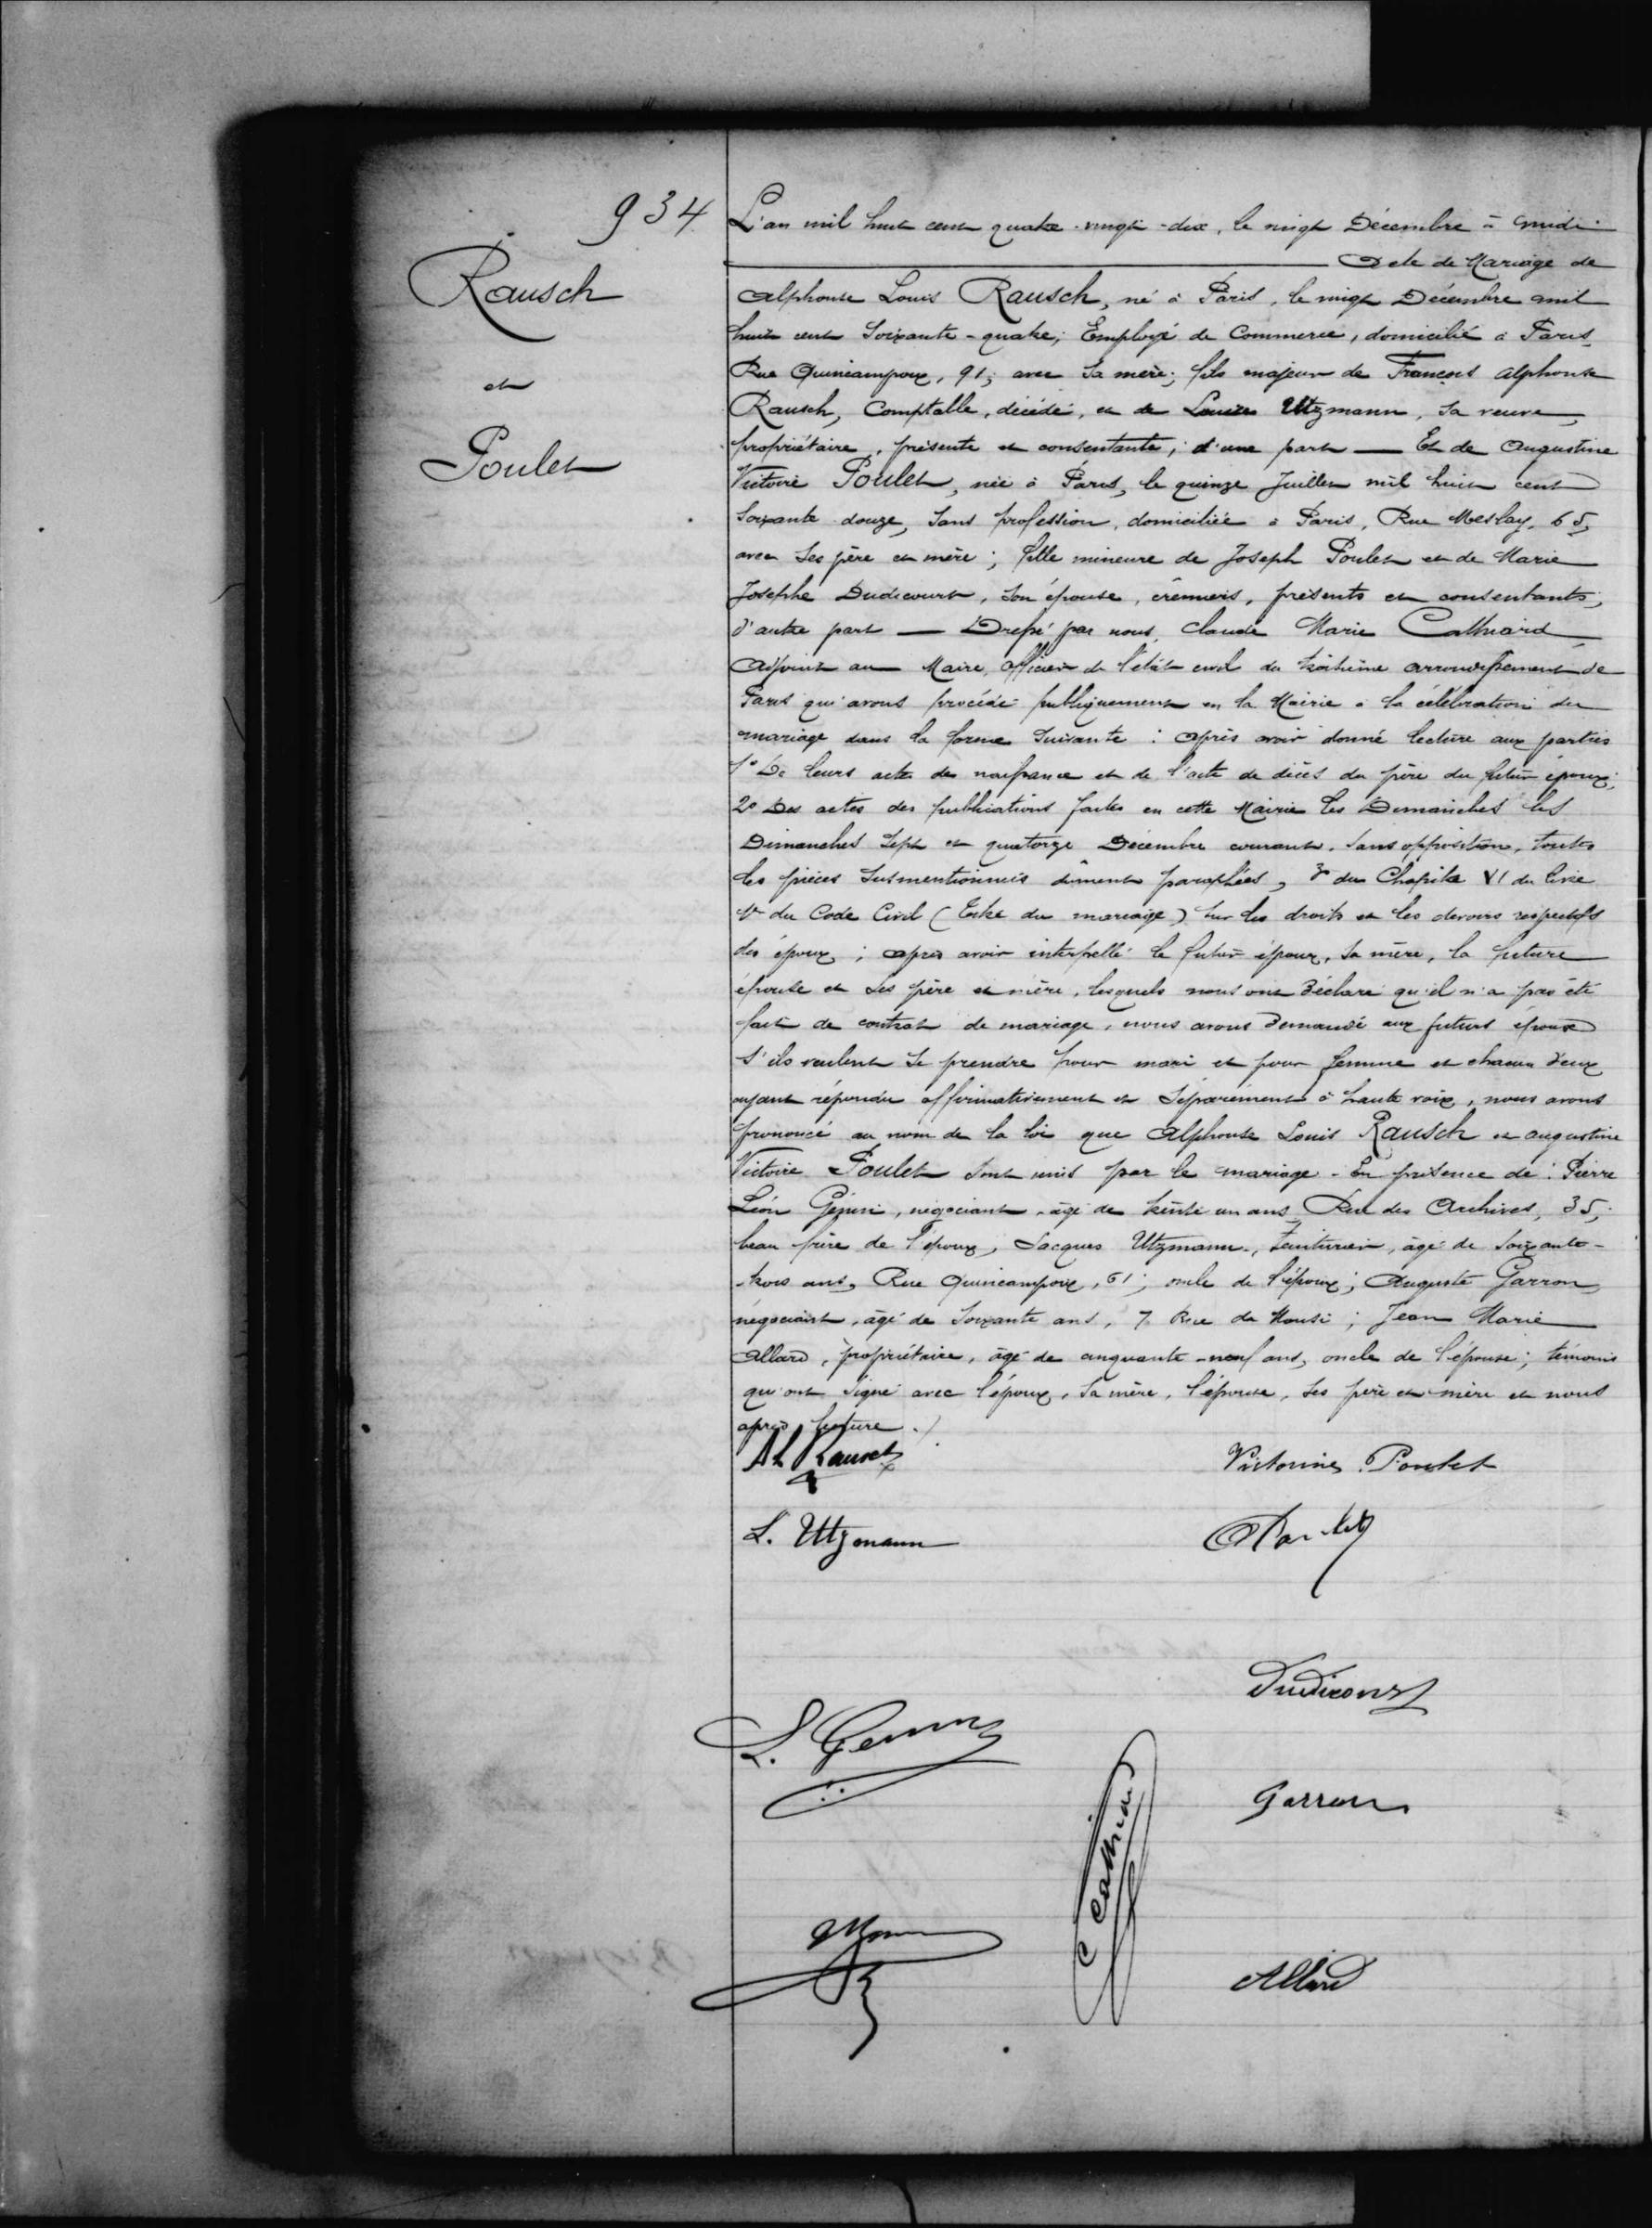934
Rausche
et
Poulet
L'an mil huit cent quatre ving-deux, le vingt Décembre à midi
-Acte de Mariage de
Alphonse Louis Rausch, né à Paris, le vingt Décembre mil
huit cent Soixante-quatre; Employé de Commerce, domicilié à Paris,
Rue Quincampoix, 91, avec sa mère; fils majur de Froment alphonse
Rausch, Comptable, décédé, et de Louise Ultzmann, sa veuve,
propriétaire, présente et consentante; d'une part-Et de Augustin
Victoire Poulet, née à Paris, le quinze Juillet mil huit cent
soixante douze, sans profession, domiciliée à Paris, Rue Meslay, 65,
avec ses père et mère; fille mineure de Joseph Poulet et de Marie
Josepha Dudecourt, son épouse, crêmiers, présents et consentants
d'autre part -Dressé par nous, Claude Marie Cathiard,
Adjoint au Maire, officier de l'état civil du troisième arrondissement de
Paris qui avons procédé publiquement en la Mairie. la célébration du
mariage dans la forme suivante: après avoir donné lecture aux parties
1° De leurs actes de naifsance et de l'acte de décès du père du futur époux
2° Des actes des publications faites en cette Mairie les Dimanches les
Dimanches sept et quatorze Décembre courant. Sans oppositions, toutes
les pièces susmentionnées dûment paraphées, 3° du Chapitre VI du livre
V du Code Civil (Titre du mariage) sur les droits et les devoirs respectifs
des époux; après avoir interpellé le futur époux, sa mère, la futur
épouse et ses père et mère, lesquels nous ont déclaré qu'il n'a pas été
fait de contrat de mariage, nous avons demandé aux futur époux
s'ils veulent se prendre pour mari et pour femme et chacun d'eux
ayant répondu affirmativement et séparèment à haute voix, nous avons
prononcé au nom de la loi que Alphonse Louis Rausch et augustine
Victoire Poulet sont unis par le mariage. En présence de: Pierre
Léon Génin, négociant, âgé de trente un ans Rue des Archives, 35;
beau père de l'époux, Jacques Uttzmam, teinturier, âgé de soixante
-trois ans, Rue Quincampoix, 61; oncle de l'époux; Auguste Garron
négociant, âgé de Soixante ans, 7 Rue de Mousi; Jean Marie
Allard, propriétaire, âgé de cinquante-neuf ans, oncle de l'épouse; témoins
qui ont signé avec l'époux, sa mère, l'épouse, ses père et mère et nous
après lecture./.
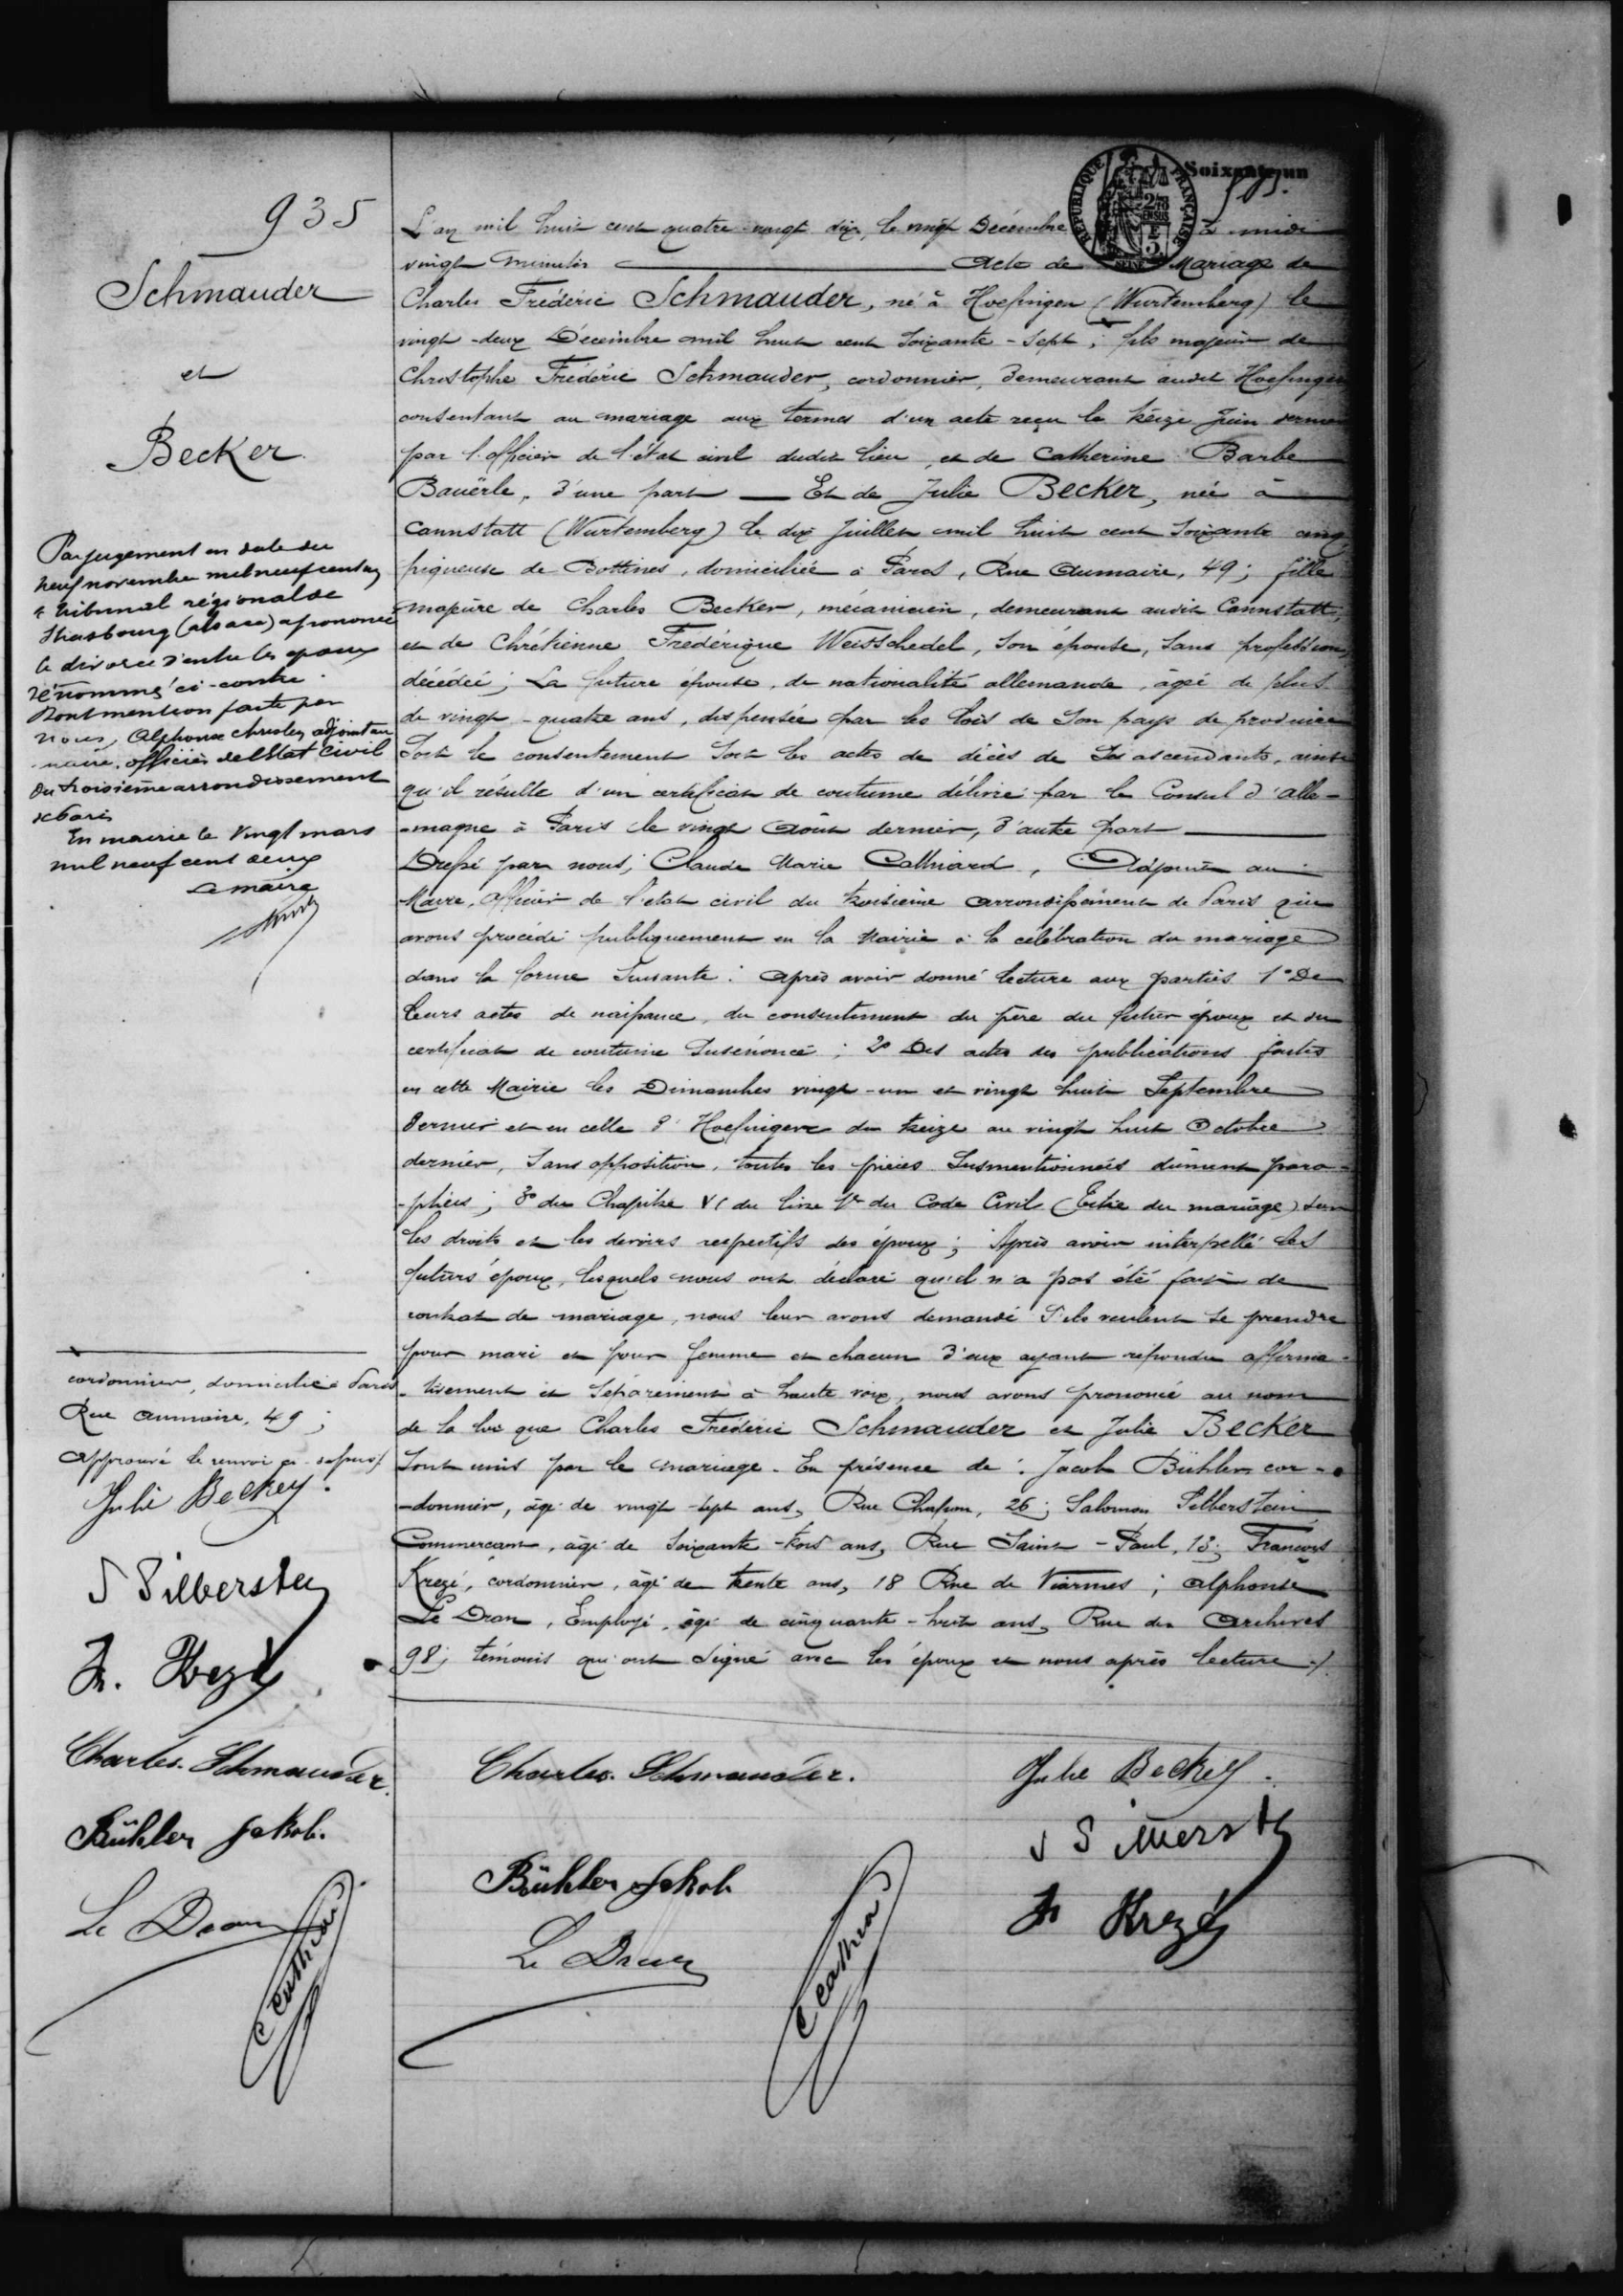935
Schmauder
et
Becker
L'an mil huit cent quatre vingt dix, le vingt Décembre à midi
vingt minutes -Acte de mariage de
Charles Frédéric Schmauder, né à Hoefinger (Wurtenberg) le
vingt deux Décmbre mil huit cent Soixante-Sept, fille majeure de
Christophe Frédéric Sohmauder, cordonnier, demeurant audit Hoefinger
consentant au mariage aux termes d'un acte reçu le treize Juin dernier
pa l'officier de l'état civil dudit lieu, et de Catherine Barbe
Bavërle, d'une part -Et de Julie Becker, née à
Connstatt (Wurtemberg) le dix Juillet mil huit cent soixxante ans
piqueuses de bottines, domiciliée à Paros, Rue Aumaire, 49; fille
majeure de Charles Becker, mécanicien, demeurant audit Connstatt,
et de Chrétienne Frédérique Weisschedel, son épouse, sans profession
décédée; La future épouse, de nationalité allemande, âgé de plus
de vingt-quatre ans, dispensée par les lois de son pays de produire
dont le consentement dont les actes de décès de Ses ascendants, ainsi
qu'il résulte d'un certificat de coutume délivré par le Conseil d'Alle-
-magne à Paris le vingt Août dernier, d'autre part -
Drefsé par nous; Claude Marie Callmard, Adjoint au
Maire, officier de l'état civil du troisième arrondissement de Paris qui
avons procédé publiquement en la Mairie à la célébration du mariage
dans la forme suivant: Après avoir donné lecture aux parties 1° De
leurs actes de naissance, du consentement du père du futur époux et du
certificat de coutume Susénoncé; 2° Des actes des publications faites
en cette Mairie les Dimanches vingt-un et vingt-juit Septembre
dernier, sans opposition, toutes les pièces susmentionnées dûment para-
-phées; 3° du Chapitre VI du livre V du Code Civil (Titre du mariage) dont
les droits et les devoirs respectifs des époux; Après avoir interpellé les
futurs époux, lesquels nous ont déclaré qu'il n'a pas été fait de
contrat de mariage, nous leur avons demandé S'ils veulent se prendre
pour mari et pour femme en chacun d'eux ayant répondu affirma
-tivement et Séparément à haute voie, nous avons prononcé au nom
de la loi que Charles Frédéric Schmauder et Julie Becker
Sont unis par le mariage. En présence de: Joseph Büller cor
-donnier, âgé de vingt-sept ans, Rue Chapon, 26: Salomon Setherstein
Commerçant, âgé de Soixante-trois ans, Rue Saint-Paul, 13; François
Krezé, cordonnier, âgé de trente ans, 18 Rue de Viarnes; Alphonse
Le Dran, Employé âgé de cinquante-huit ans, Rue des Archives
98; témoins qui ont signé avec les époux et nous après lecture./.